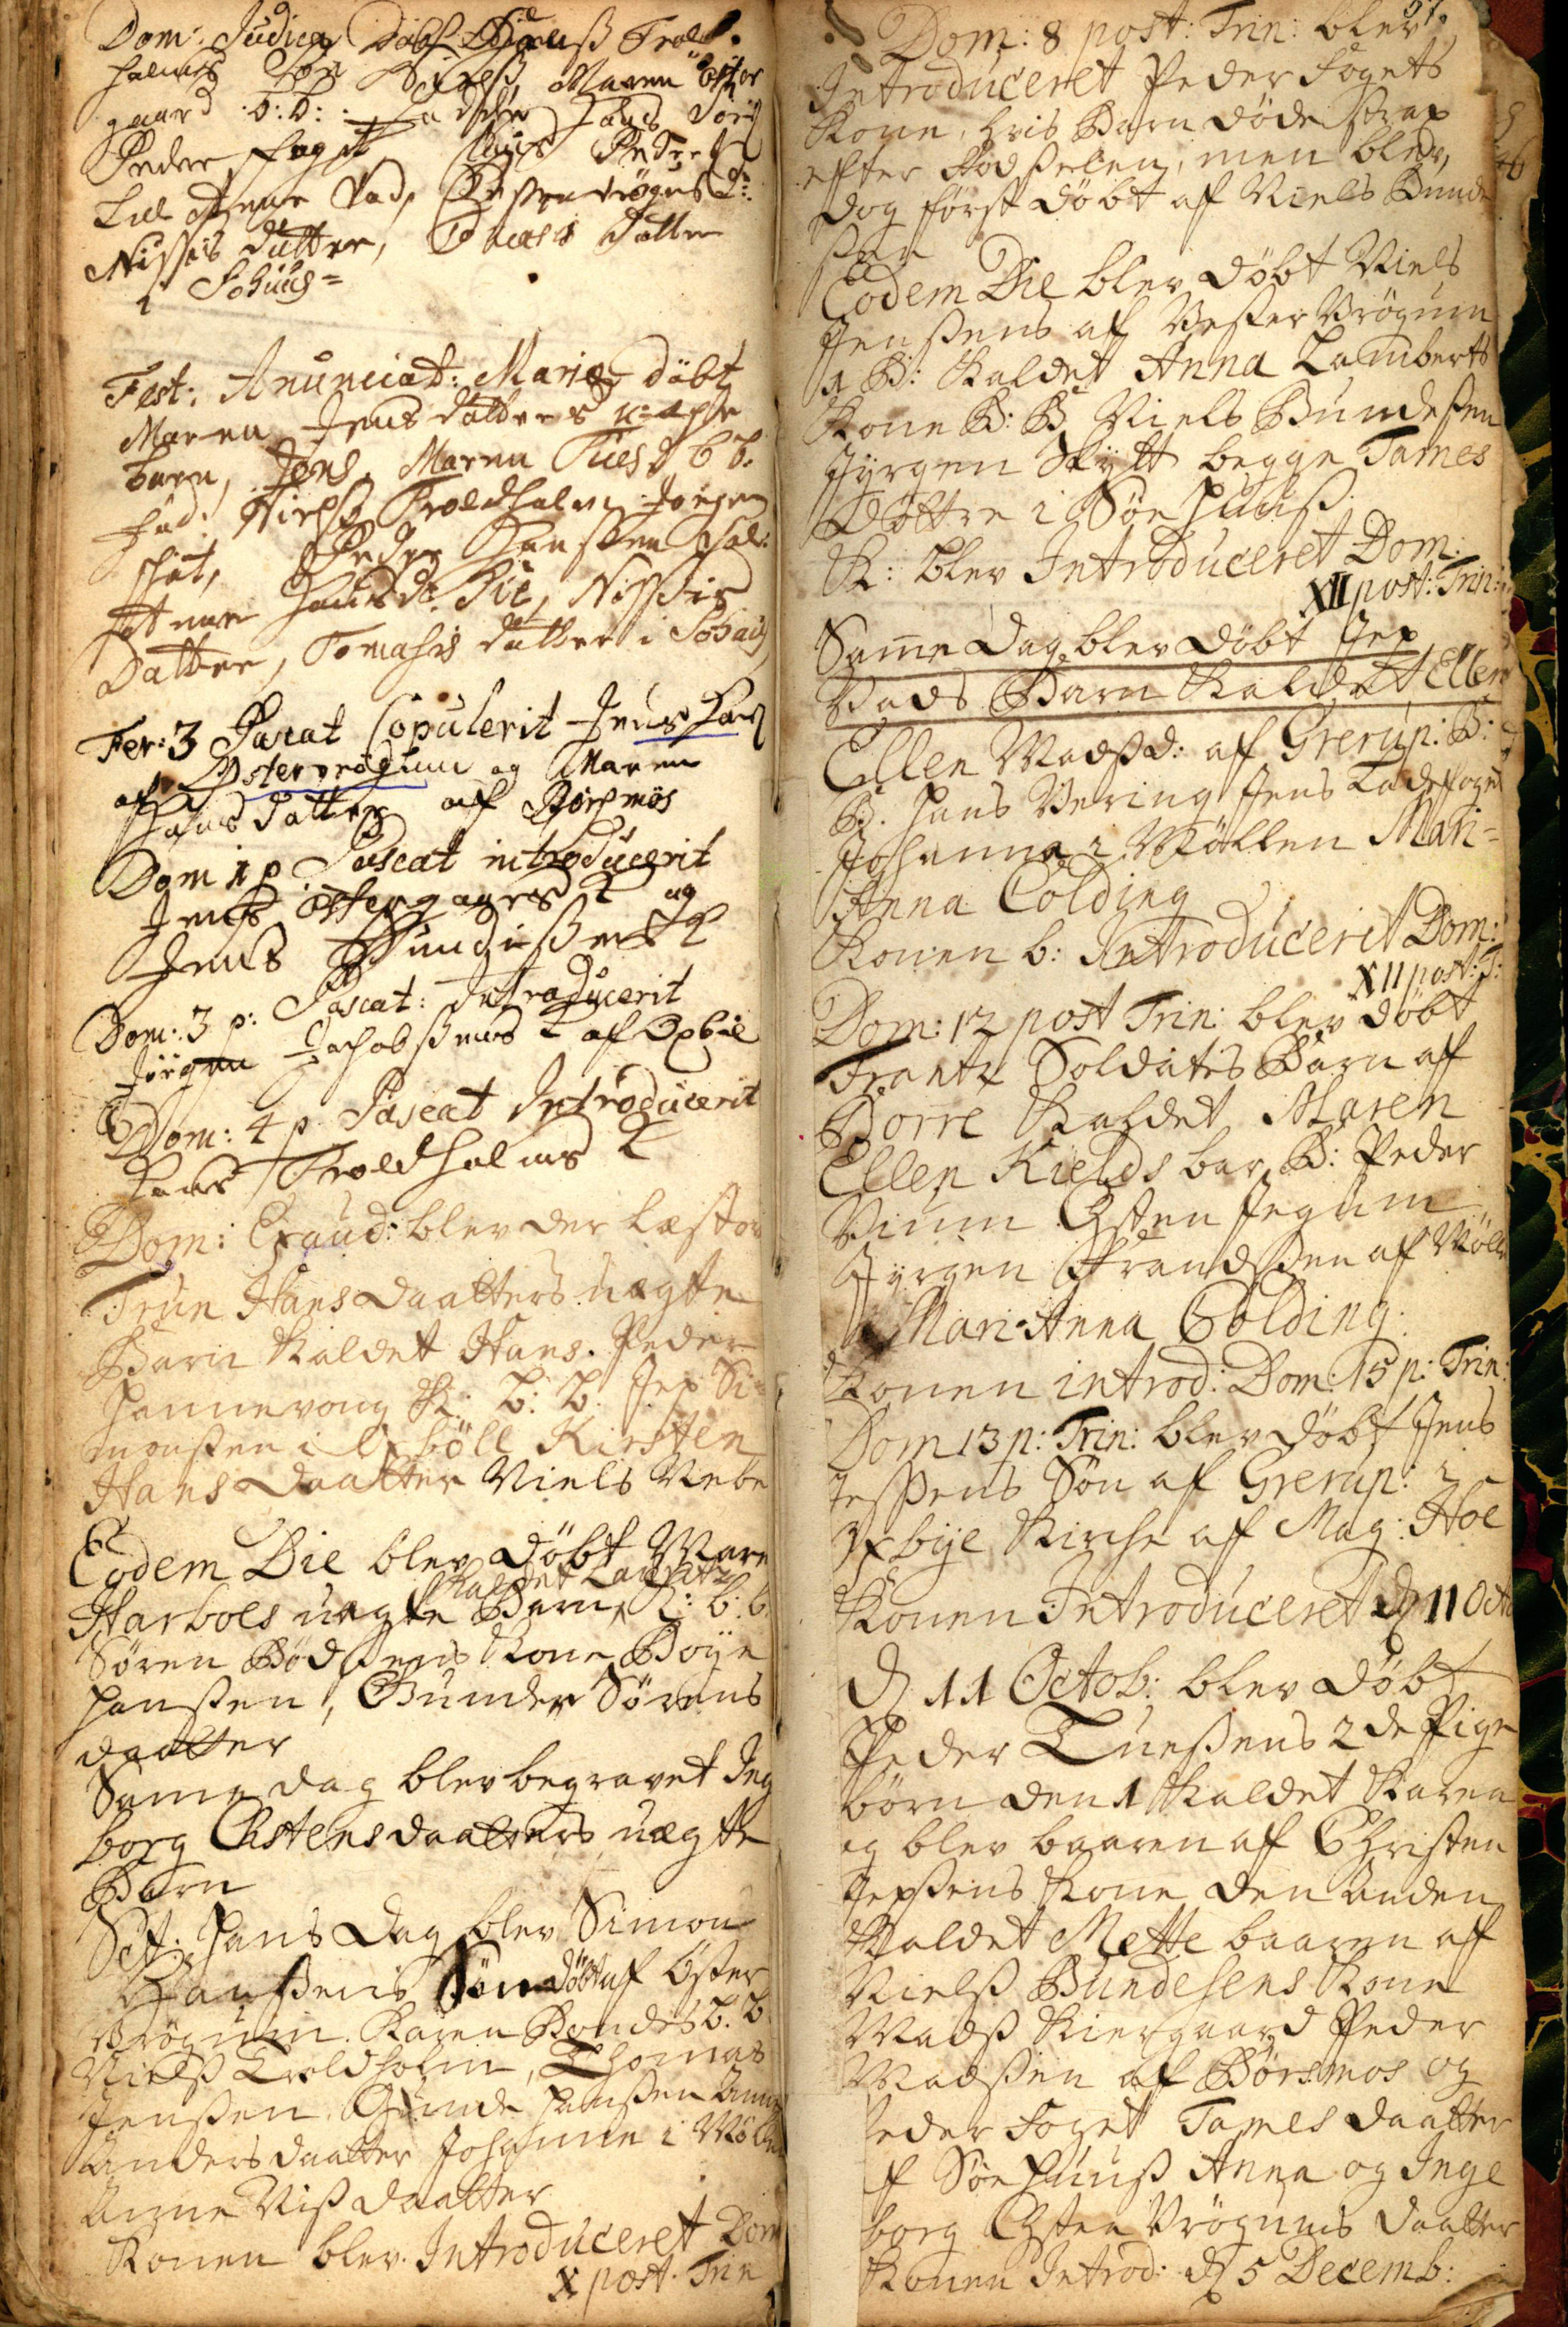Dom: Judica Døbt Hans Trold¬
holms Søn Niels, Maren Øster
gaard b:b: Fadde Hans Søres
Peder Foget Claus Peders
Lille Anne Vad, Christen Vrøgums dr
Nissis Datter, Tomasis Datter
i Søhuus=
Fest: Anunciat: Mariæ Døbt
Maren Jensdatters u=æchte
Barn, Jens. Maren Tuesd bb:
Fad: Niels Troldholm, Jørgen
Schøt, Peder Hansen Sol.
Anne Hansdr hic##, Nissis
Datter, Tomasis Datter i Søhuus
Fer: 3 Pascat Copulerit Jens Hans
af Østervrøgum og Maren
Hansdatter af Børsmøs
Dom 1 p Pascat introducerit
Jens Østergaars K og
Jens Bundisens K
Dom: 3 p: Pascat: Introducerit
Jørgen Jachobsens K af Oxbøl
Dom: 4 p Pascat Introducerit
Hans Troldholms K
Dom: Exaud: blev der læst ov
Trun Hansdaatters uægte
Barn kaldet Hans. Peder
Hannevangs K: b: b. Jep Si¬
monsen i Oxbøll, Kirsten
Hansdaatter Niels Nebel
Eodem Die blev døbt Maren
kaldet Lauritz
Harboes uægte Barn K: b:b.
Søren Bødsens Kone Boye
Hansen, Gunder Sørens
daatter
Samme Dag blev begravet Ing
borg Christensdaatters uægte
Barn
Sct: Hans Dag blev Simon
Hansens Søn døbt af Øster
vrøgum Karen Bondes b. b.
Niels Troldholm, Thomas
Jensen, Gunde Hansen Anne
Andersdaatter Johanne i Møllen
Anne Nisdaatter. 
Konen blev Introduceret Dom
X post. Trin
57.
Dom: 8 post: Trin: blev
Introduceret Peder Fogets
Kone, hvis Barn døde strax
efter Fødselen, men blev,
dog først døbt af Niels Bunde
sen
Eodem Die blev døbt Niels
Jensens af Vestervrøgum
1 B. kaldet Anna Lamberts
Kone B:B Niels Bundesen
Jyrgen Skytt begge Tames
Døttre i Søehuus
K. blev Introduceret Dom:
XII post: Trin:
Samme Dag blev døbt Jep
Vads Barn kaldet Ellen
Ellen Madsd: af Grerup: B:
B. Hans Vering, Jens Ladefoget
Johanna i Møllen, Mari¬
Anna Colding
Konen b: Introduceret Dom:
XII post: T:
Dom: 12 post Trin: blev døbt
Frantz Soldats Barn af
Borre kaldet Maren
Ellen Kields bar B: Peder
Vium Christen Jegum
Jyrgen Frandsen af Møllen
Mari-Anna Colding
Konen introd: Dom: 15 p: Trin:
Dom13 p: Trin:  blev døbt Jens
Jessens Søn af Grerup: i
Oxbye Kirche af Mag: Hoe
Konen Introduceret d 11 Oct
D 11 Octob: blev døbt
Peder Tuesens 2de Pige
børn den 1 kaldet Karen
og blev baaren af Christen
Jepsens Kone den Anden
Kaldet Mette baaren af
Niels Bundesens Kone
Mads Kiergaard Peder
Madsen af Børsmos og
Peder Foget Tames Daatter
f Søehuus, Anna og Inge
borg Christen Vrøgums Daattere
Konen Introd; d 5 Decemb:
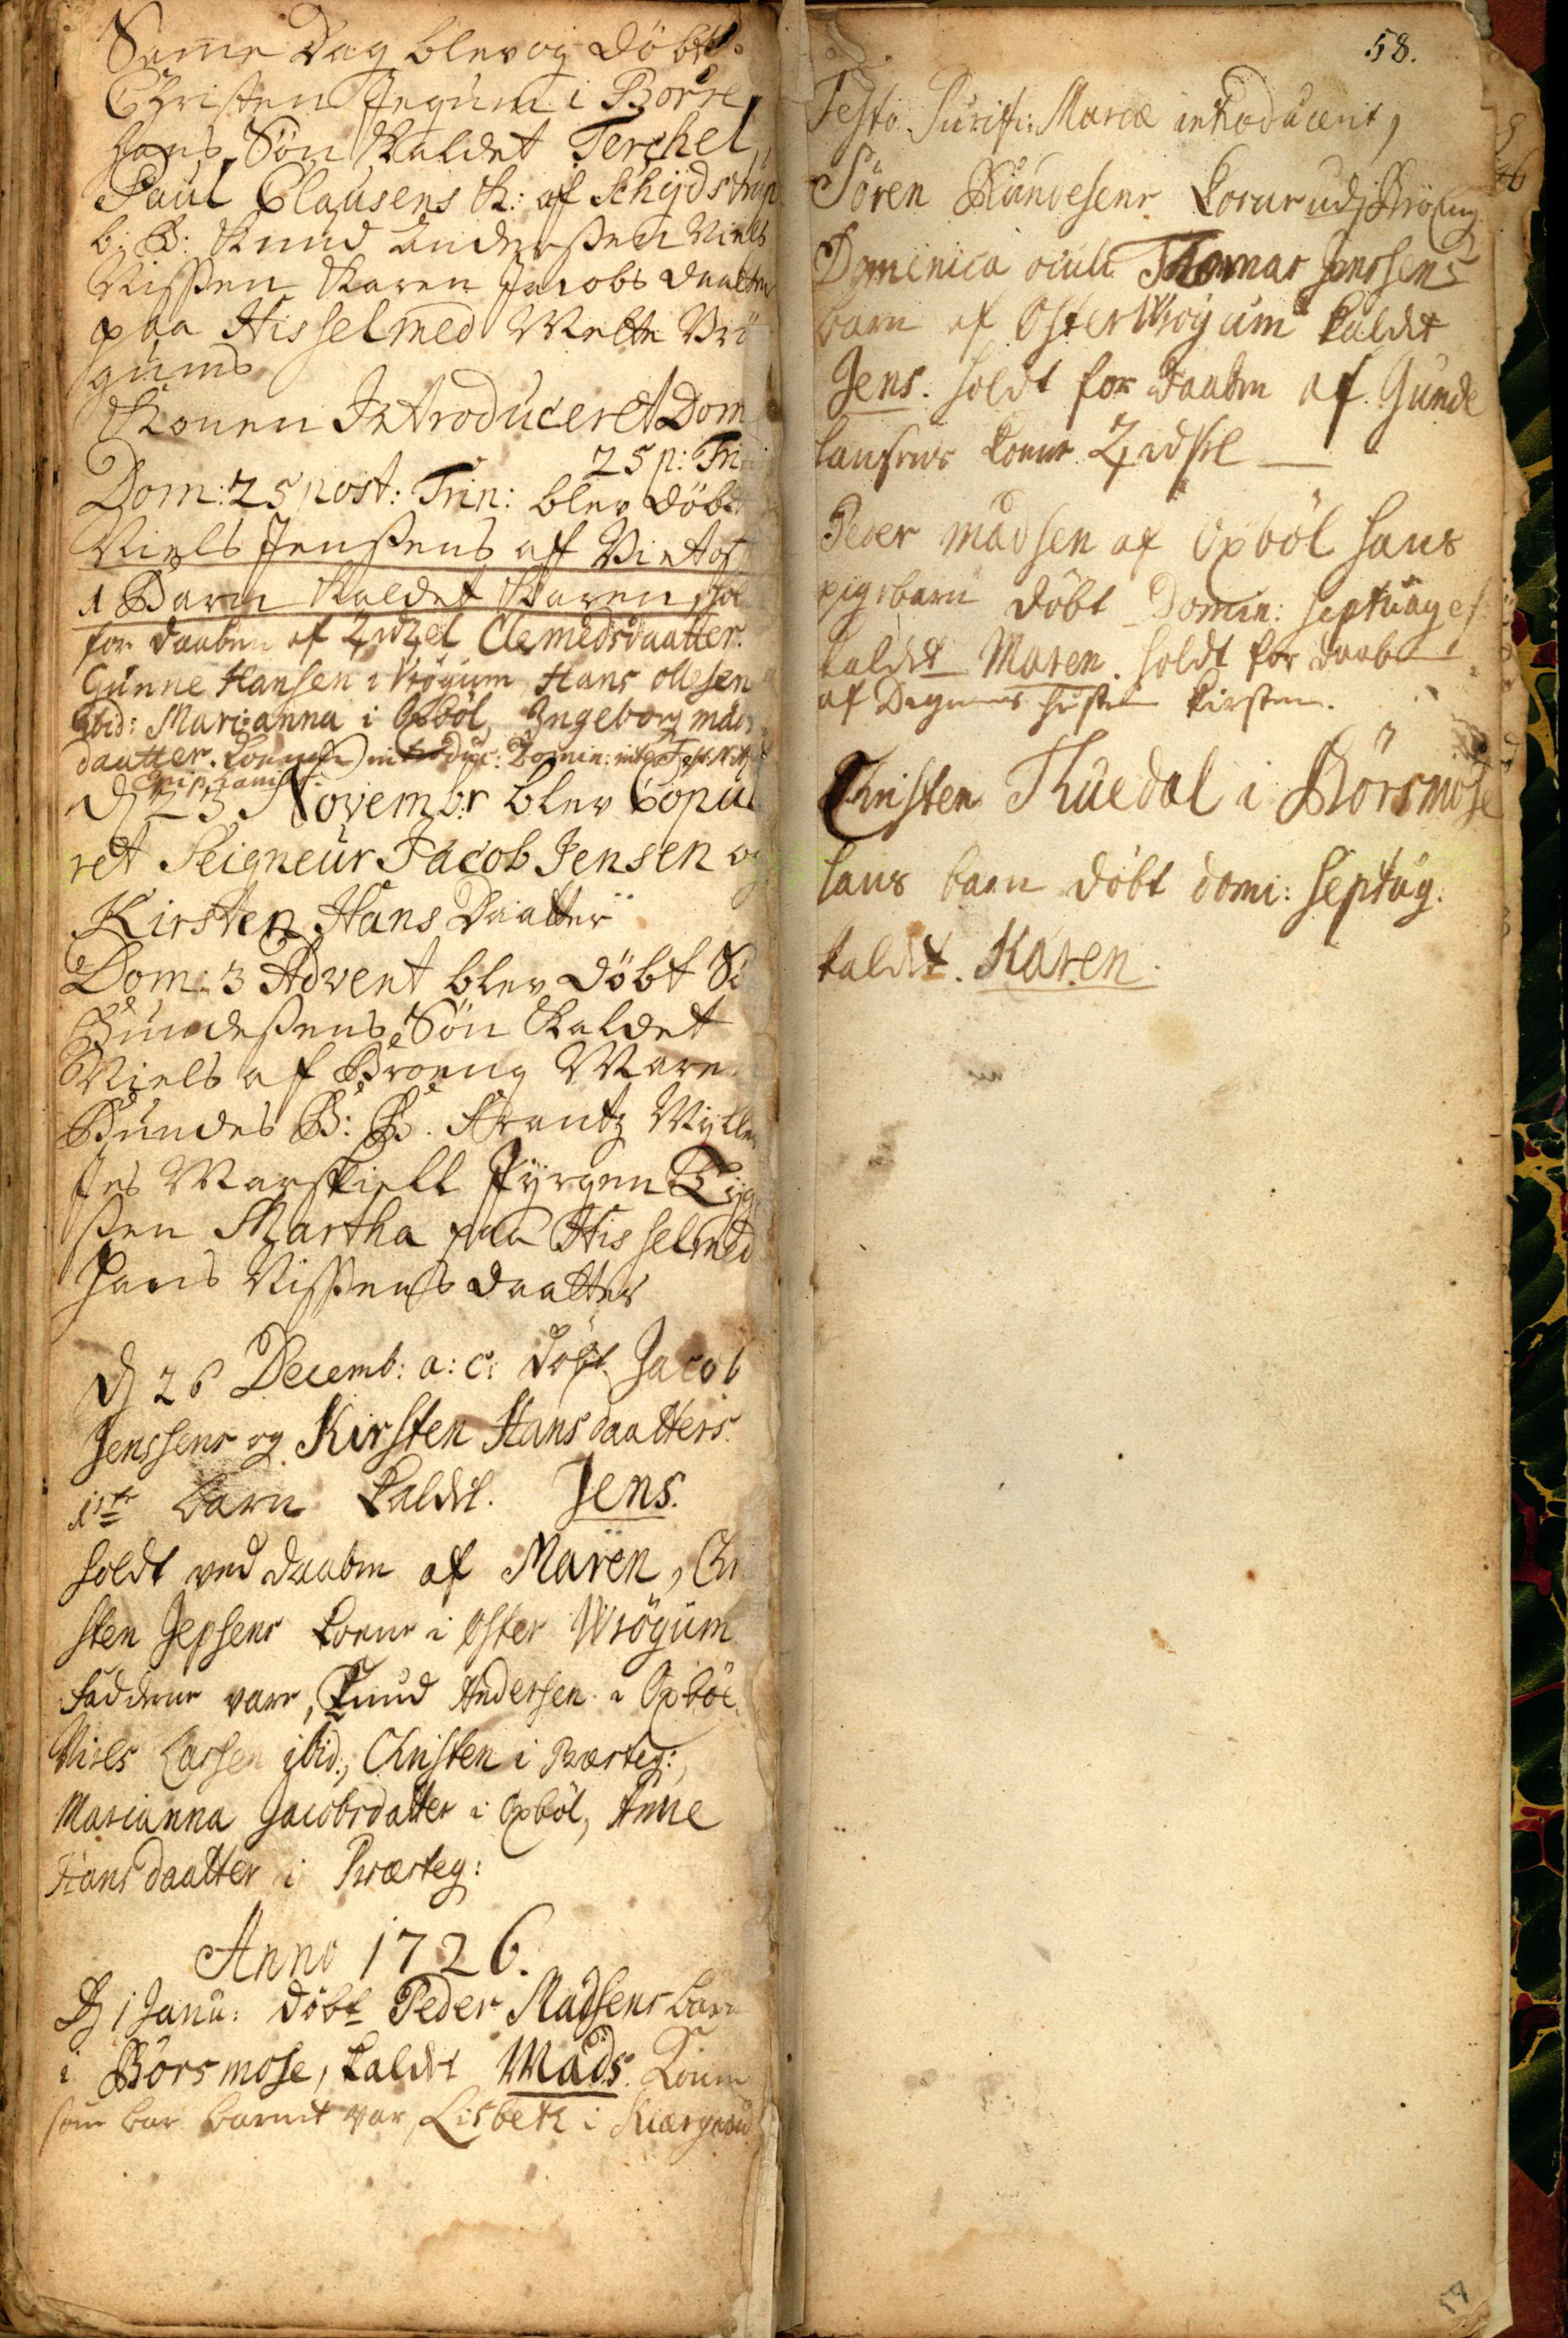Samme Dag blev døbt
Christen Jegum i Borre
hans Søn kaldet Terchel
Paul Clausens K: af Schydstrup
b: B: Knud Andersen Niels
Nissen Karen Jacobsdaatter
paa Hisselmed Mette Vrø
gum
Konen Introduceret Dom
25 p: Tri
Dom: 25 post: Trin: blev døbt
Niels Jensens af Vietoft
1 Barn kaldet Karen, hol
for Daaben af Zidzel Clemedsdaatter.
Gunne Hansen i Vrøgum, Hans Ollesen
ibid: Mari:anna i Oxbøl, Ingeborg Mads¬
daatter. Konen introduc: Domin: inter Fest: NA
Epiphanius##
D 23 Novemb:r blev Copul
ret Seigneur Jacob Jensen og
Kirsten Hans Daatter
Dom 3 Advent blev døbt Sø
Bundesens Søn kaldet
Niels af Broeng Maren
Bundes B: B. Frantz Myller
Jes Marskiell, Jyrgen Tyg¬
sen Martha paa Hisselmed
Hans Nissens Daatter
D 26 Decemb: a: c: døbt Jacob
Jensens og Kirsten Hansdaatters.
1ste Barn kaldt Jens.
holdt ved Daaben af Maren, Chr
sten Jepsens Koene i Øster Wrøgum
Faddere vare Knud Andersen i Oxbøl
Niels Lassen ibid: Christen i Præsteg:
Marianna Jacobsdatter i Oxbøl, Anne
Hansdaatter i Præsteg:
Anno 1726.
D 1 Janu:. døbt Peder Madsens Barn
i Børsmose, kaldt Mads. Konen
som bar Barnet var Lisbeth i Kiærgaard
58
57
Festo Purif: Mariæ introduceret,
Søren Bundesnes Koene udi Brøeng
Dominica oculi Thomas Jensens
Barn af Øster Wrøgum kaldt
Jens. holdt for Daaben af Gunde
Hansens Koene Zidsel
Peder Madsen af Oxbøl hans
pigebarn døbt Domin: Septuages:
kaldt Maren. holdt for Daab
af Degnens Hustrue Kirsten.
Christen Thuedal i Børsmose
hans Barn døbt Domi: Septug.
kaldt Karen